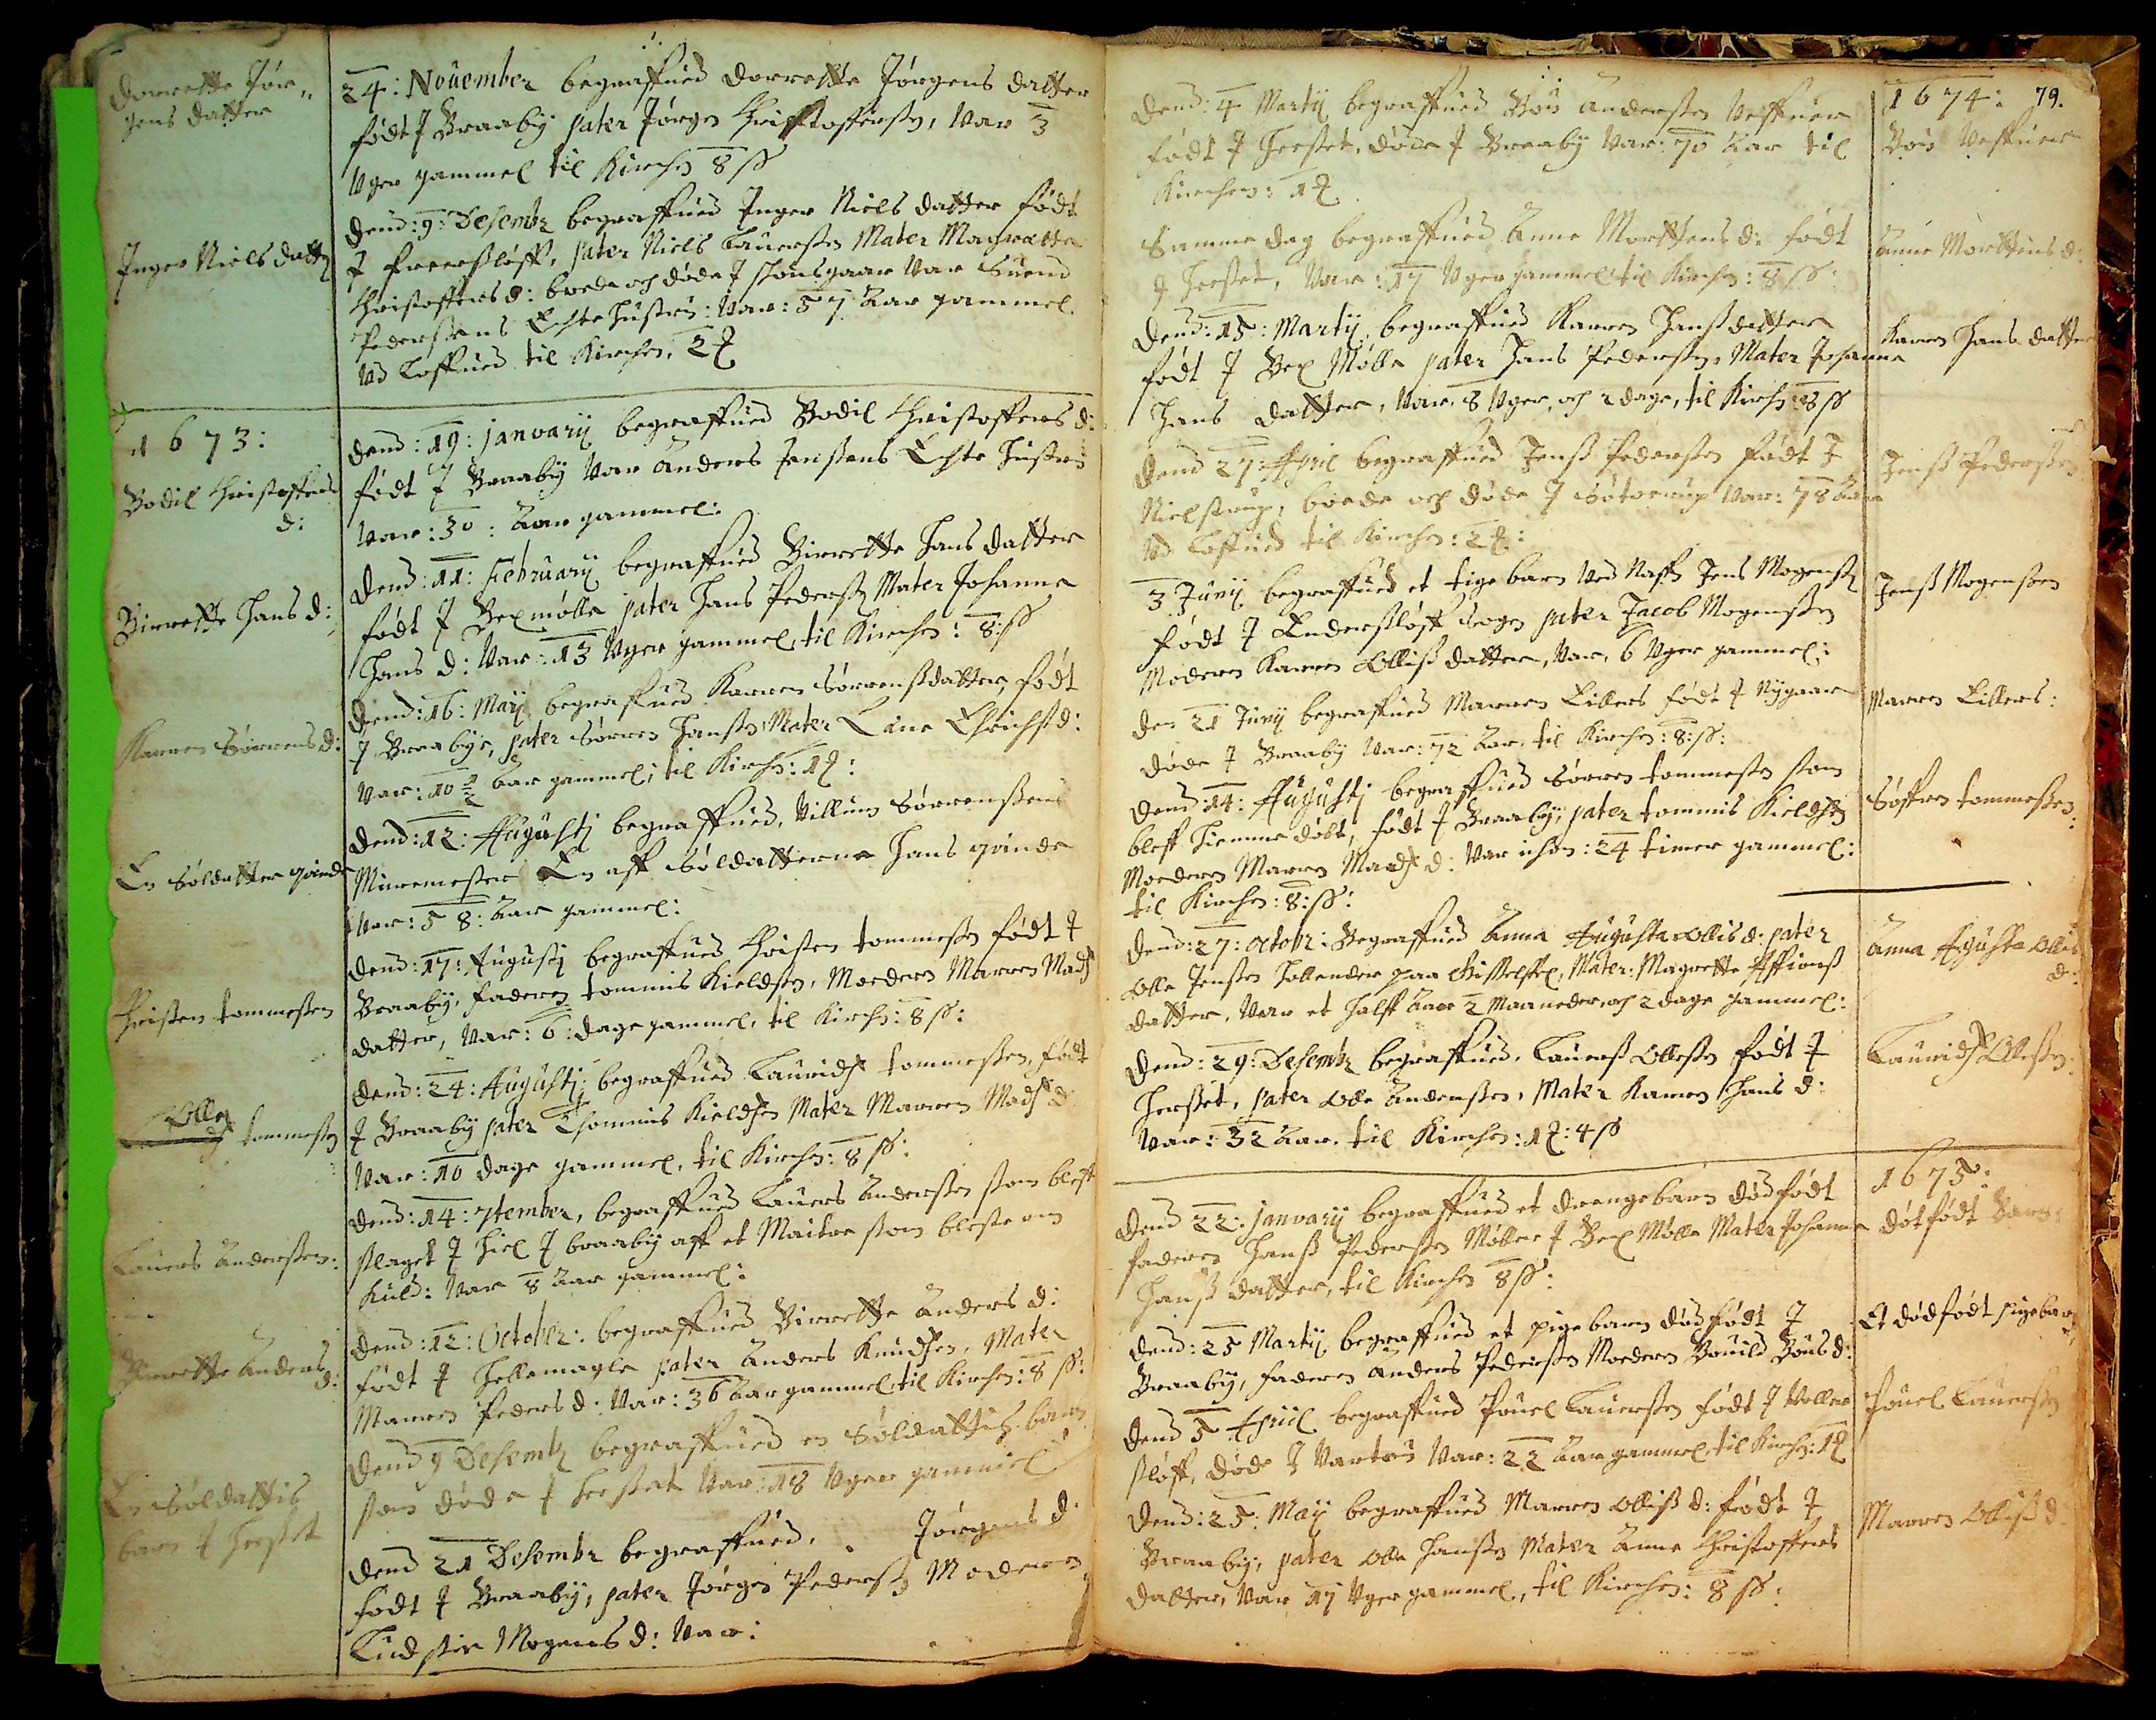Dorrette Jør¬
gensdatter
24: Nouember begraffued Dorrette Jørgensdatter
født J Braaby, Pater Jørgen Christoffersen. Vare 3
Uger gammel, til Kirchen 8 Sk. 
Jnger Nielsdatter
Dend: 9: Desember begraffued Jnger Nielsdatter født
J Freersløff. Pater Niels Lauersen Mater Margaretta
Christoffersd: boede och døde J Skousgaar: Vare Suend
Pedersens Echte hustru: Vare: 57 Aar gammel,
Udloffued til Kirchen 2 Mk.
1673:
Bodil Christoffers
d:
Dend: 19: January begraffued Bodil Christoffers d:
født J Braaby, Vare Anders Jensens Echte hustrue.
Vare: 30: Aar gammel:
Birrette Hans d:
Dend: 11: February begraffued Birrette Hansdatter
født J Bexmølle, Pater Hans Pedersen, Mater Johanna
Hans d: Vare: 13 Uger gammel, til Kirchen: 8: Sk
Karren Sørrens d:
Dend: 16: May begraffued Karren Sørrensdatter, født
J Braabye, Pater Sørren Hansen. Mater Læne Ehrichsd:
Var: 10 ## Aar gammel, til Kirchen: 1 Mk:
En Søldatter quinde
Dend: 12: Augusty begraffued Villum Sørrensens
Murmesters En aff Søldatterne Hans quinde.
Var: 58: Aar gammel:
Christen Tommesen
Den: 17: Augusty begraffued Christen Tommesøn født J
Braaby, Faderen Tommis Kieldsen, Moderen Marren Mads
datter. Var: 6: Dage gammel, til Kirchen: 8 Sk:
Olle Laurids
Tommesen
Den: 24: Augusty begraffued Laurids Tommesen, født
J Braaby, Pater Thommis Kieldsen, Mater Marren Mads D.
Var: 10 Dage gammel, til Kirchen: 8 Sk:
Lauers Andersøn
Dend: 14: 7tember, begraffued Lauers Andersen som blef
slaget i hiel J Braaby af et Maitre## som bleste om
kuld: Var 8 Aar gammel:
Birrette Anders d:
Dend: 12: October: begraffued Birrette Anders d:
født J Hellemagle, Pater Anders Knudsen, Mater
Marren Peders D: Var 36 Aar gammel, til Kirchen: 8 Sk:
En Søldattis
barn i Hirset
Dend 9 Desembr begraffued en Søldattis Barn
som døde J Hirsit. Vare 18 Uger gammel
Dend 21 Decembr begraffued,
Jørgens D.
født J Braaby, Pater Jørgen Pedersen, Moderen
Ludsie Mogens d: Var:
Dend 4. Marty begraffued Bou Andersen Veffuer
født J Herset, døde J Braaby. Var 70 Aar, Til
Kirchen 1 Mk.
79.
1674:
Bou Veffuer
Samme dag begraffued Anna Morthensd:## født
J Hirset. Vare: 17 Uger gammel, til Kirchen: 8 Sk.
Anna Morthensd:##
Dend: 15: Marty begraffued Karen Hansdatter
født J Bex Mølle, Pater Hans Pedersen, Mater Johanne
Hans datter. Var 8 Uger och 2 Dage, til Kirchen 8 Sk
Karen Hansdatter
Dend 27: April begraffued Jens Pedersen født J
Nielstrup boede och døde J Søtoerup. Var: 78 Aar
Ud Loffued til Kirchen: 2 Mk:
Jens Pedersen
3. Juny begraffued et tigebarn ved Naffn Jens Mogensen
født J Endersløff sogn, Pater Jacob Mogensen
Moderen Karen Ollisdatter. Var 6 Uger gammel:
Jens Mogensen
Den 21 Juny begraffued Marren Eillers født J Nygaard
døde J Braaby. Var: 72 Aar, til Kirchen: 8: Sk:
Marren Eillers:
Dend 14: Augusti begraffued Sørren Tommesen som
bleff Hiemmedøbt, født i Braaby, Pater Tommis Kieldsøn
Moderen Maren Mads d: Var iche##: 24 Timer gammel:
Til Kirchen: 8: Sk:
Søffren Tommesen
Dend: 27: Octobr: begraffued Anna Augusta Ollisd: Pater
Olle Jensøn Hollænder paa Gisselfel: Mater: Margarette Affions
datter. Var et halft Aar 2 Maaneder, och 2 Dage gammel:
Anna Augusta Ollis
d:
Den: 29 Desembr. begraffued, Lauers Ollesen født J
Hersit, Pater Olle Andersen, Mater Karren Hans d:
Var: 32 Aar, til Kirchen: 1 Mk: 4 Sk
Laurids Ollesen:
Dend 22: January begraffued et Drengebarn dødfødt,
Faderen Hans Pedersen Møller J Bex Mølle, Mater Johanne
Hansdatter, Til Kirchen 8 Sk:
1675
Dødfødt Dreng:
Dend: 25 Marty begraffued et Pigebarn dødfødt J
Braaby, Faderen Anders Pedersen, Moederen Bouild Bousd:
Et dødfødt Pigebarn
Dend 5 Apriil begraffued Pouel Lauersen født J Ullers##
sløff døde i Vartov. Var: 22 Aar gammel, til Kirchen: 1 Mk.
Pouel Lauersen
Dend: 25: May begraffued Marren Ollis d: født J
Braaby, Pater Olle Hansen, Mater Anna Christophers
datter, Var 17 Uger gammel, til Kirchen: 8 Sk:
Marren Ollis d:
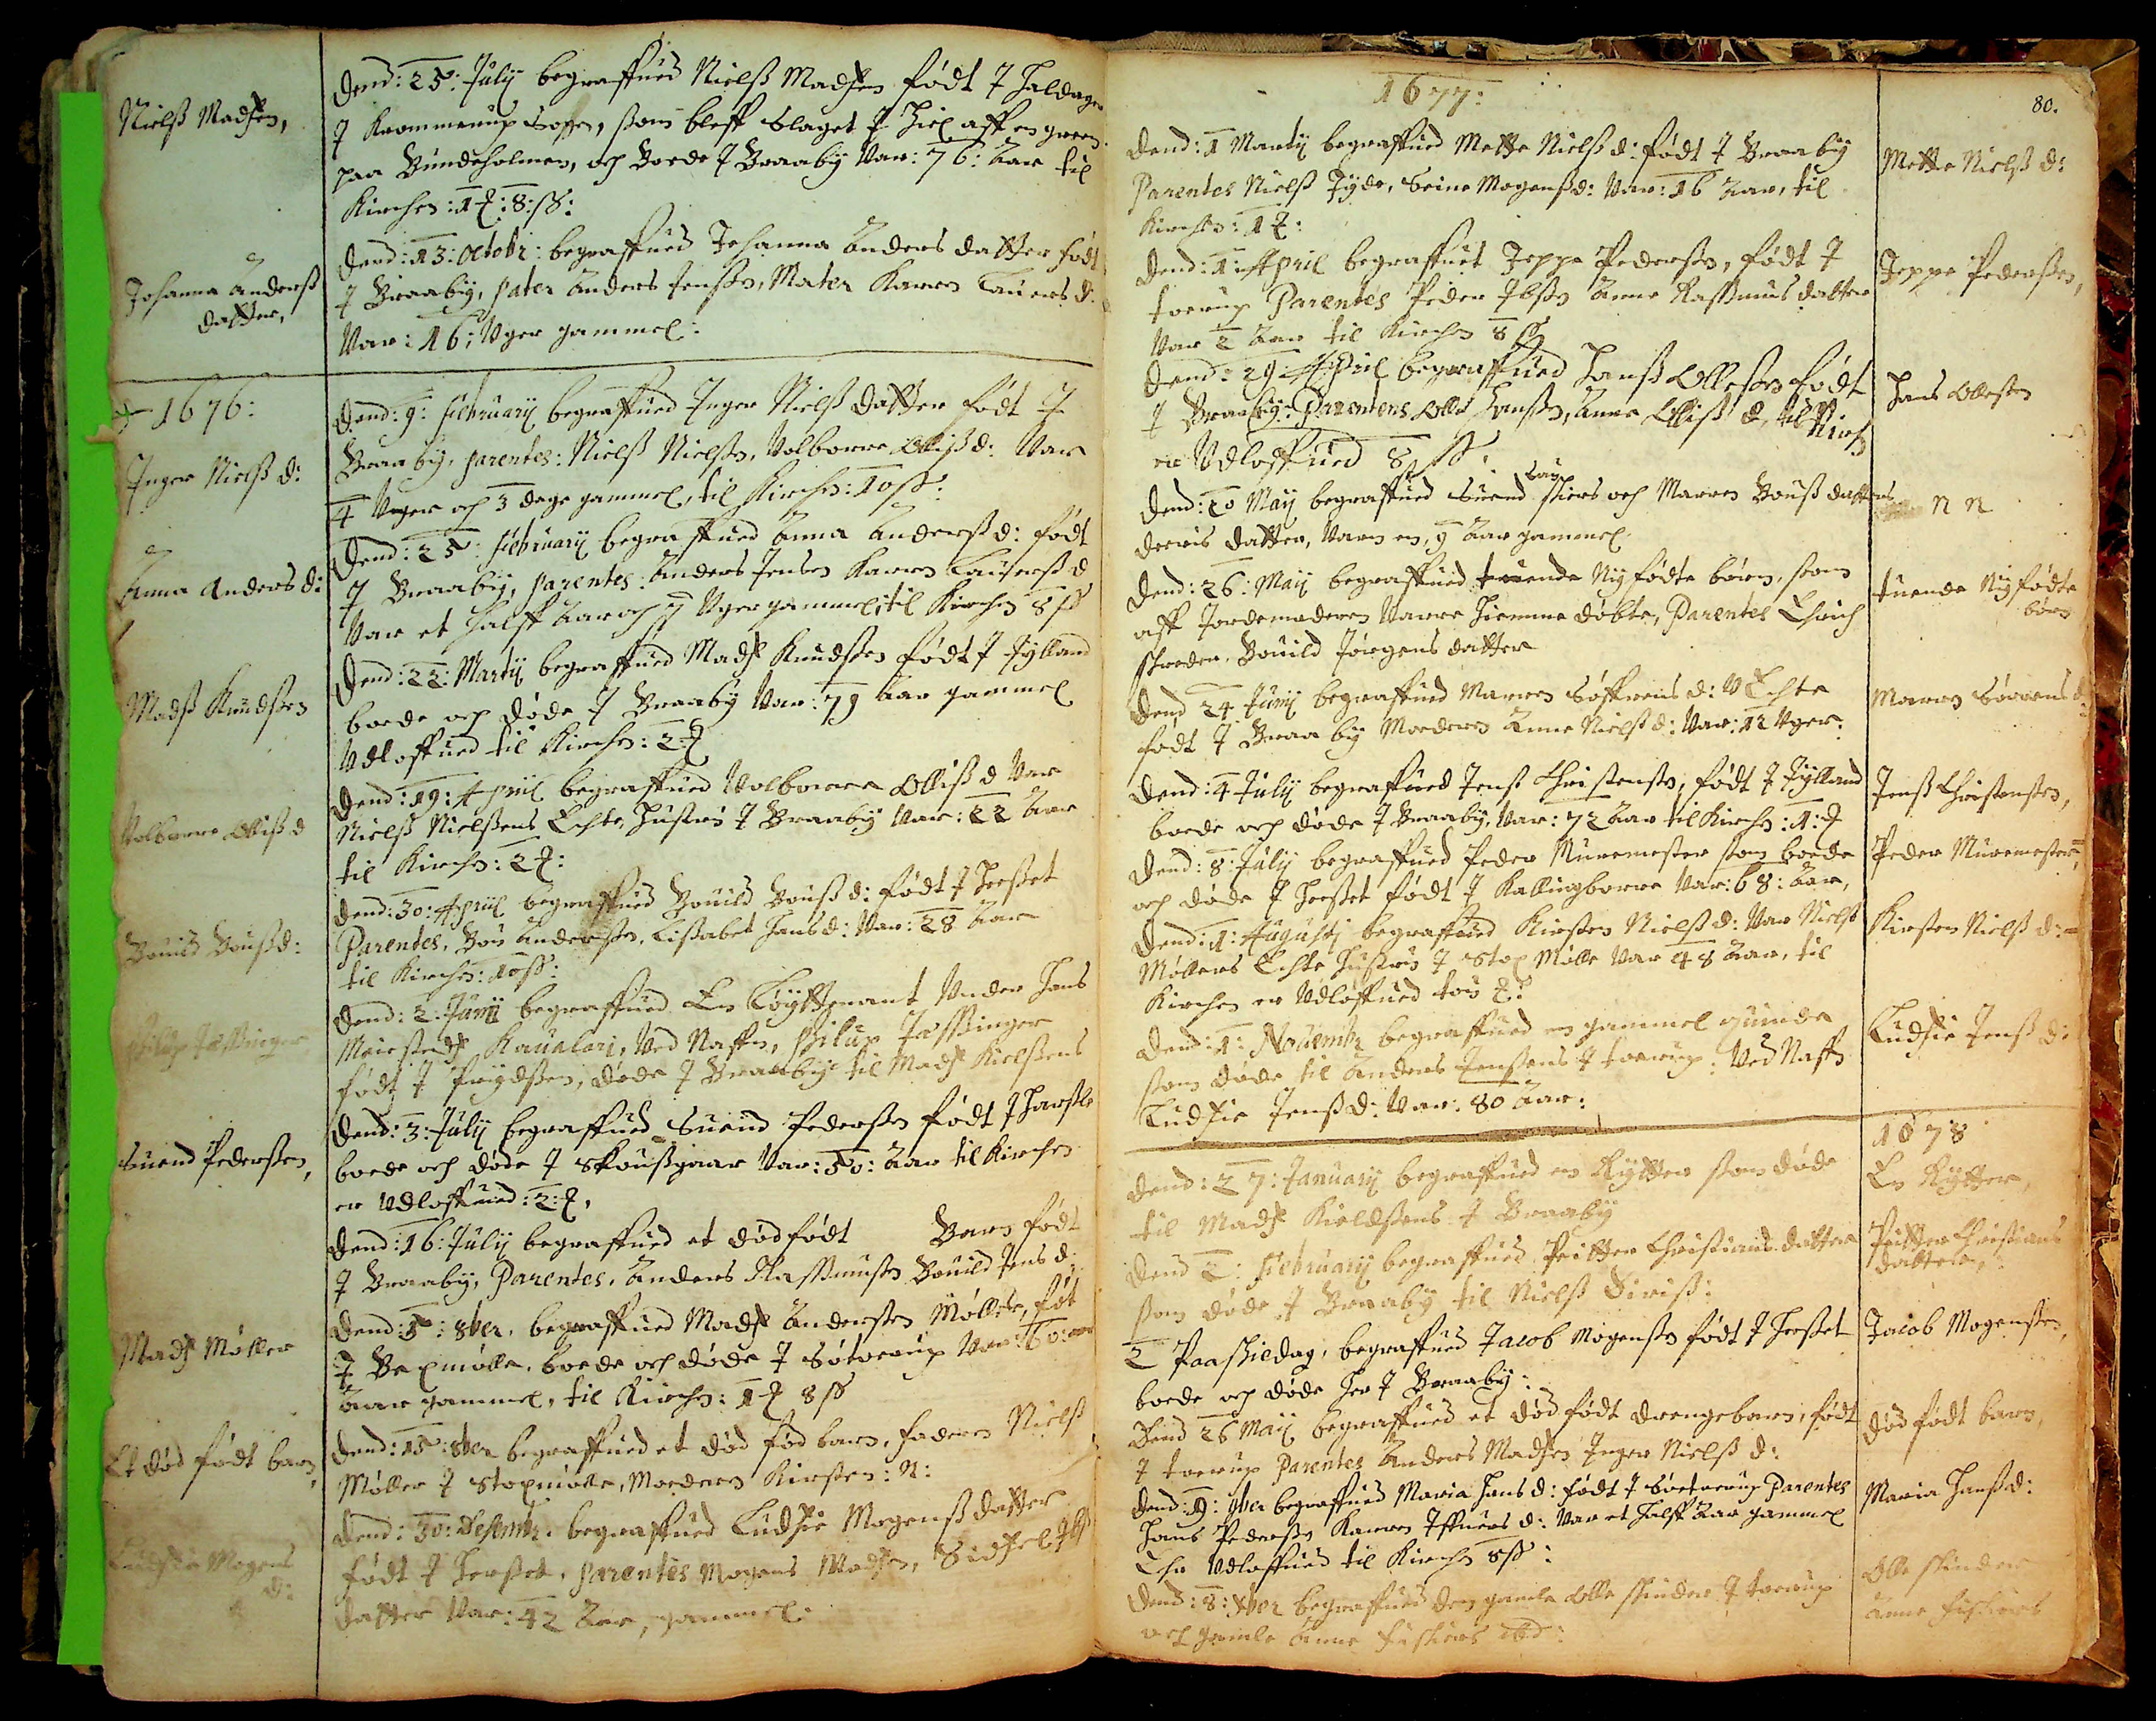Niels Madsen
Dend: 25: July begraffued Niels Madsen født J Heldager##
J Krummerup## Sogn## som bleff slaget i hiel aff en gren##
paa Bonde##Holmen och boede J Braaby. Var: 76: Aar, til
Kirchen: 1 Mk: 8: Sk:
Johanne Anders
datter,
Dend: 13: Octobr: begraffued Johanna Anders datter, født
J Braaby, Pater Anders Jensen, Mater Karren Lauers d.
Var: 16: Uger gammel:
1676:
Jnger Niels d:
Dend: 9: February begraffued Jnger Niels datter født J
Braaby, Parentes: Niels Nielsen, Valborre Ollisd: Var
4 Uger och 3 Dage gammel, til Kirchen: 10 Sk:
Anna Anders d:
Dend: 25: February begraffued Anna Anders d: født
J Braaby, Parentes Anders Jensen, Karren Lauers d
Var et halft Aar och 7 Uger gammel. Til Kirche 8 Sk.
Mads Knudsen
Dend: 22: Marty begraffued Mads Knudsen født J Jylland
boede och døde J Braaby. Var: 79 Aar gammel
Udloffued til Kirchen: 2 Mk.
Valborre Ollis d:
Dend: 19: Apriil begraffued Valborre Ollis d., Var
Niels Nielsens Echte hustrue J Braaby. Var: 22 Aar,
til Kirchen: 2 Mk.
Bouild Bousd:
Dend: 30: Apriil begraffued Bouild Bousd: født J Herset,
Parentes. Bou Andersen, Lissabet Hansd: Var: 28 Aar,
til Kirchen: 10 Sk:
Philup## Jæssinger
Dend: 2. Juny begraffued En Løÿttenant Under Hans
Maiesteds Kaualari. Ved Naffn Philup Jæssinger
født J Prydsen, døde J Braaby til Mads Kielsen.
Suend Pedersen
Dend: 3: July begraffued Suend Pedersen Født J Harsle
boede och døde J Skousgaar. Var: 50 Aar, til Kirchen
er Udloffued :2 :Mk.
Dend: 16: July begraffued et dødfødt
Barn født
J Braaby, Parentes. Anders Rasmussen, Bouild Jens d.
Mads Møller
Dend: 5: 8ber begraffued Mads Andersen Møller føt
J Bexmølle, boede och døde J Søtorup. Var: 60 ##
Aar gammel. Til Kirchen: 1Mk 8 Sk.
Et død født barn
Dend: 15: 8ber begraffued et død fød barn, Fader Niels
Møller J Stoxmølle, Moederen Kirsten: N:
Ludsie Mogens
d:
Dend: 30: Desembr. begraffued Ludsie Mogensdatter
født J Herset, Parentis Mogens Madsen, Sidsel Jbs
datter. Var: 42 Aar, gammel:
1677
Dend: 1. Marty begraffued Mette Nielsd. født J Braaby,
Parentes Niels Jyde, Seine Mogensd: Var: 16 Aar, til
Kirchen: 1 Mk.
80.
Mette Nielsd:
Dend: 1: April begraffuit Jeppe Pedersøn, født J
Toerup, Parentes Peder Jbsen, Anna Rasmusdatter.
Var 2 Aar, til Kirchen 8 Sk.
Jeppe Pedersen
Dend: 29: April begraffued Hans Ollesøn, født
J Braaby, Parentes Olle Hansen, Anna Ollisd., til Kirchen
er Udloffued 8 Sk.
Hans Ollesøn
Lauer
Dend: 20 May begraffued Suend## søn## och Marren Bousdatter
deris datter. Var en, 9 Aar gammel.
NN
Dend: 26: May begraffued tuende Ny fødte børn, som
aff Jordemoderen varre Hiemme døbte, Parentis Ehrich
Skreder, Bouild Jørgensdatter.
Tuende Ny fødte
børn
Dend 24. Juny begraffued Maren Søffrensd: Uechte
fød J Braaby, Moederen Anna Nielsd: Var: 12 Uger:
Maren Sørrensd:
Dend: 4 July begraffued Jens Christensen, født J Jylland,
boede och døde J Braaby. Var: 72 Aar, til Kirchen: 1: Mk.
Jens Christensøn
Dend: 8: July begraffued Peder Muremester, som boede
och døde J Herset, født J Kalungborre. Var 68: Aar.
Peder Muremester
Dend: 1: Augusti begraffued Kirsten Nielsd: Var Niels
Møllers Echte hustrue, J Stoxmølle. Var 48 Aar, til
Kirchen er Udløffued toe Mk:
Kirsten Nielsd:
Dend: 1: Nouember begraffued en gammel Quinde
som døde til Anders Jensøns J Toerup: Ved Naffn
Ludsie Jensd: Var: 80 Aar:
Ludsie Jensd:
Dend: 27: January begraffued en Rytter som døde
til Mads Kieldsøns J Braaby.
1678
En Rytter
Dend: 2: February begraffued Peitter Christians datter
som døde J Braaby til Niels Diris:
Peitter Christians
datter 
2 Paaskedag begraffued Jacob Mogensen, født J Herset
boede och døde her i Braaby:
Jacob Mogensen
Dend 26 May begraffued et dødfødt Drengebarn, født
J Toerup, Parentes Anders Madsen, Inger Niels d:
død født barn
Dend: 9: 9ber. begraffued Maria Hansd: født J Søetoerup. Parentes
Hans Pedersen, Karren Jffursd: Var et Halft Aar gammel,
Ehr Udloffued til Kirchen 8 Sk:
Maria Hansd:
Dend: 18: Xber. begraffued Den gamle Olle Skinder## J Toerup
och gamle Anna Fiskirs ibd:
Olle Skinder##
Anne Fiskiers##